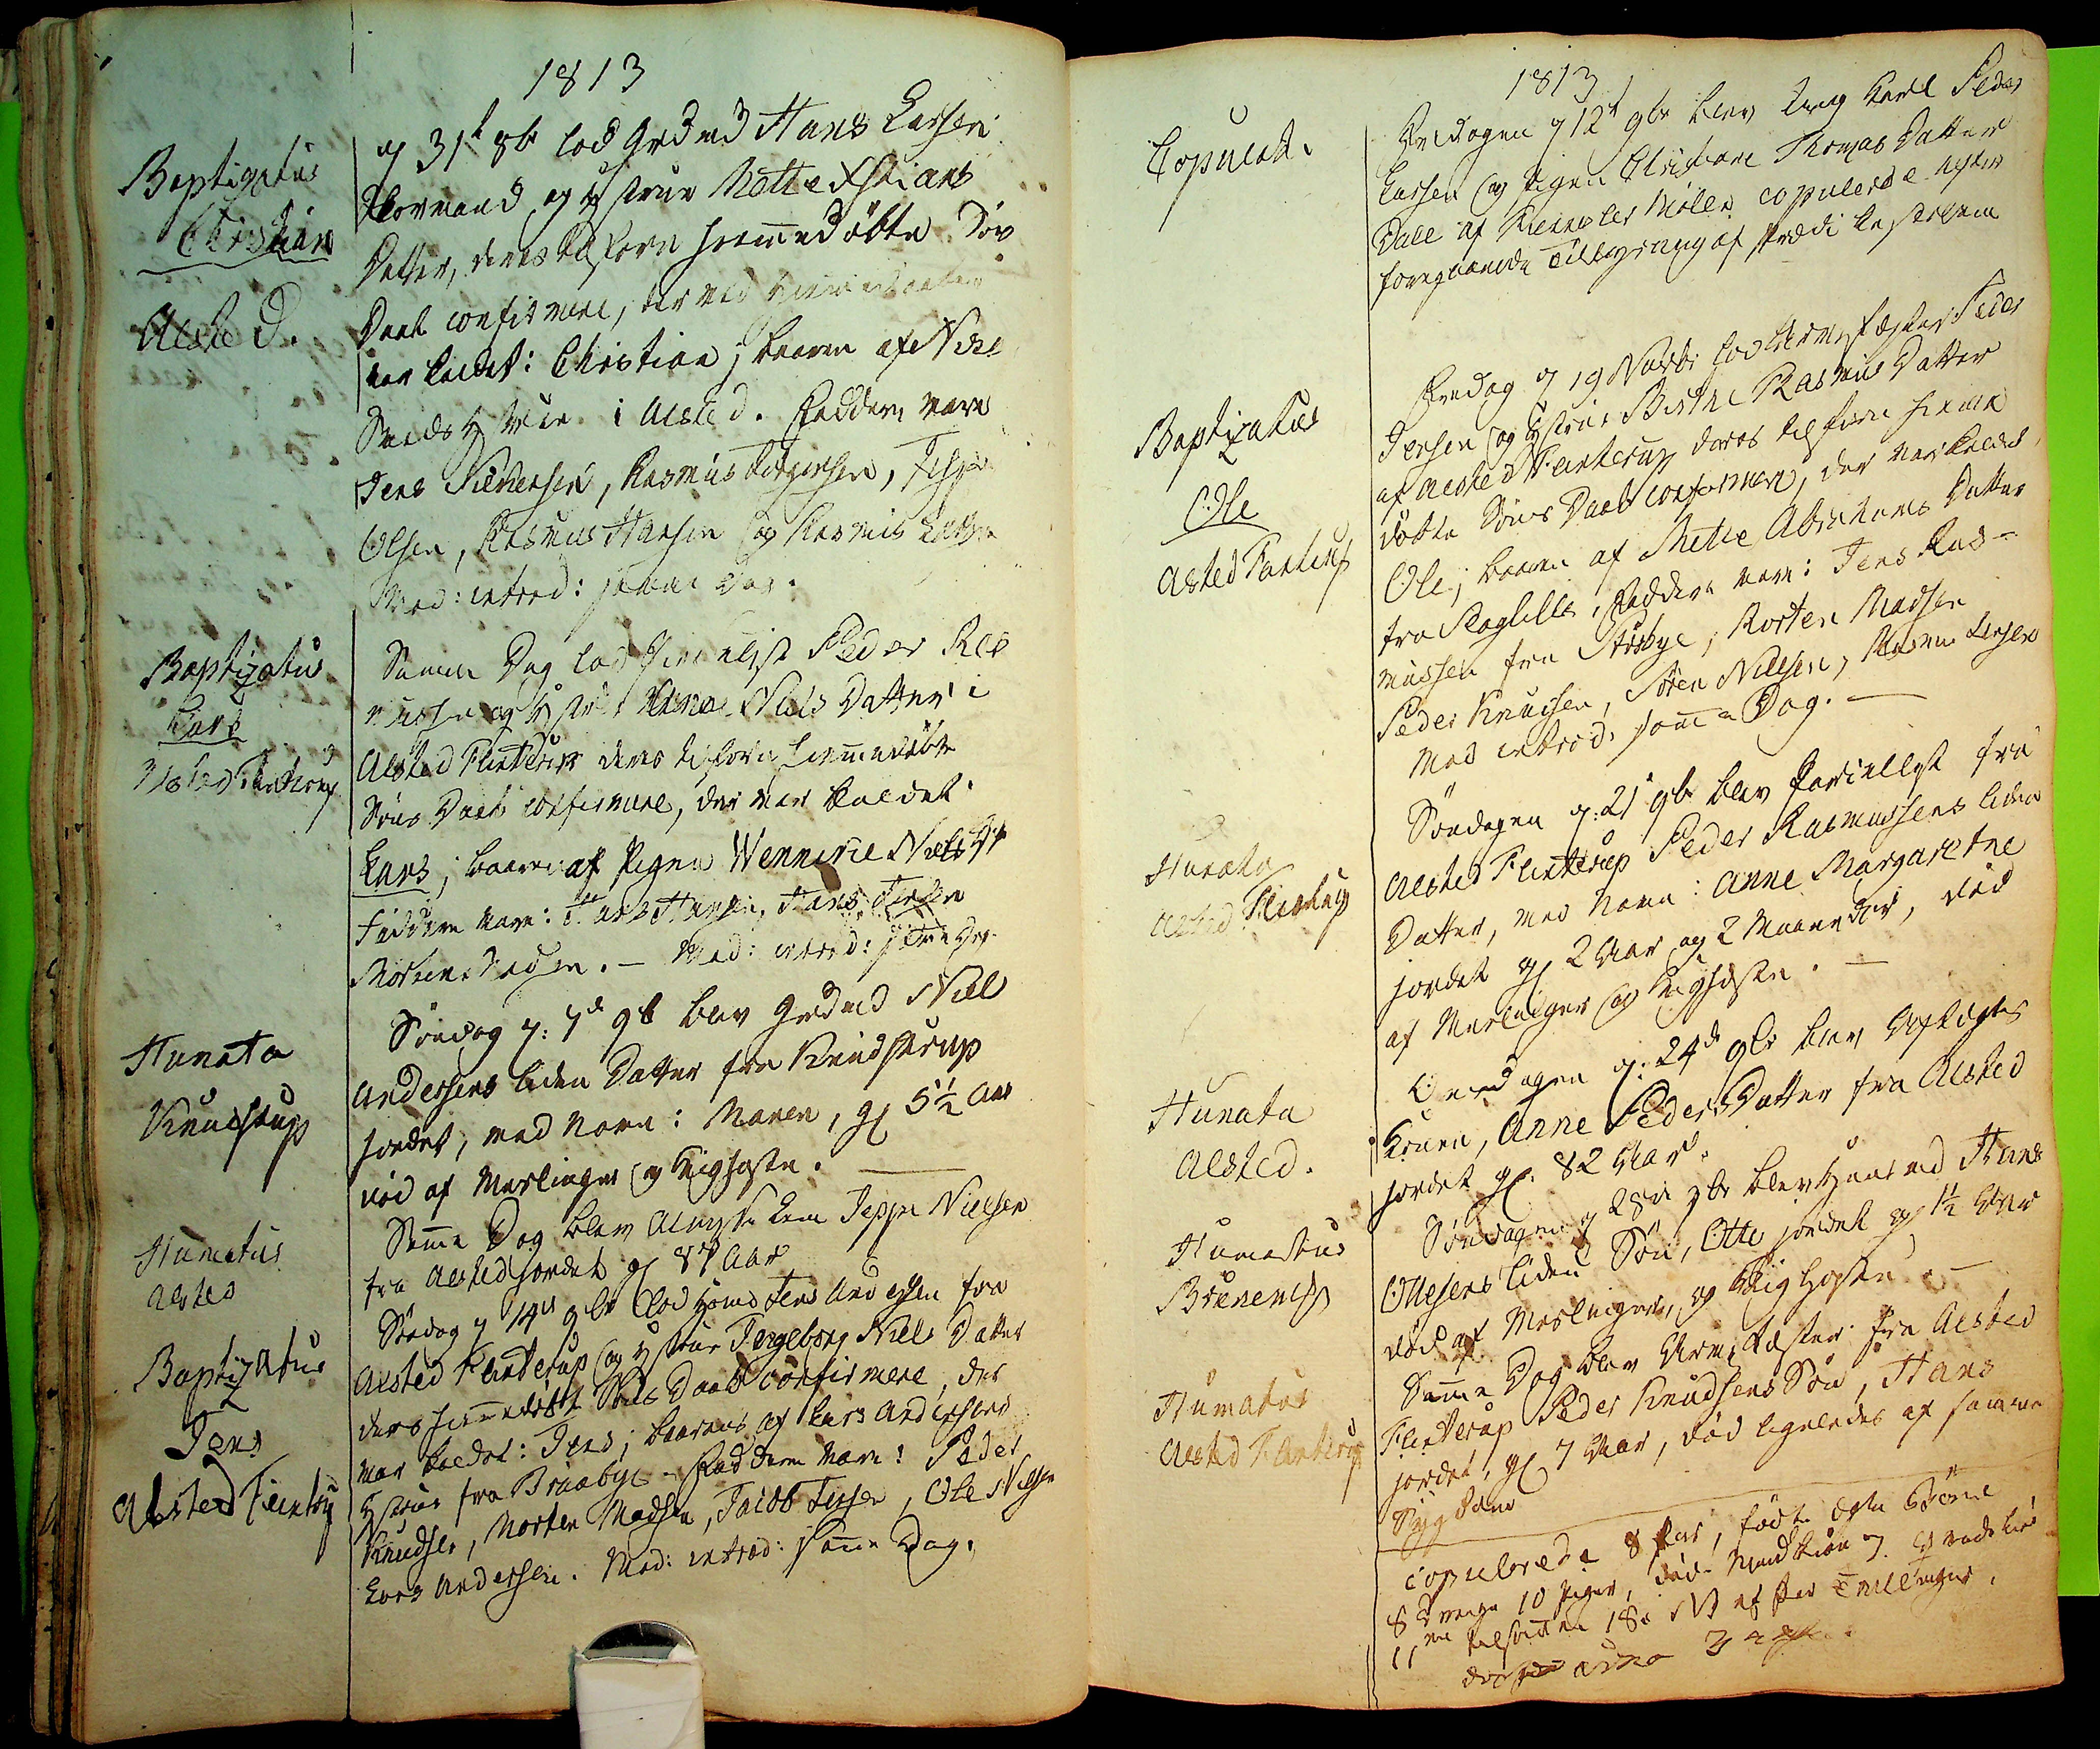Baptizatus
Christian
Alsted.
Baptizatus
Lars
Alsted Flinterup
Humata
Knudstrup
Hamatus
Alsted
Baptizatus
Jens
Alsted Flinterup
1813
d 31te 8br lod Grdmd Hans Larsen
Skovmand og Hstrue Mette Xstians
Datter, deres tilforn hiemmedøbte Søns
Daab confirmere, der ved Hiemmedaaben
var kaldet: Christian; baaren af Niels
Smeds Hstrue i Alsted. Faddere vare
Jens Silkensen, Rasmus Jørgensen, Jesper
Olsen, Rasmus Hansen og Rasmus Larsen
Mod: introd: samme Dag
Samme Dag lod Parcelist Peder Ras¬
missen og Hstrue Anna Niels Datter i
Alsted deres tilforn hiemmedøbte
Søns Daab confirmere, der var kaldet
Lars, baaren af Pigen Wenneke Niels Dtr
Faddere vare: Hans Hansen, Hans Jensen
Morten Madsen. - Mod: introd: samme Dag.
Søndag d: 7de 9br blev Grdmd Niels
Andersens liden Datter fra Knudstrup
jordet, ved Navn: Maren, gl: 5½ Aar
død af Meslingert og Kighoste.
Samme Dag blev Almisse Lem Jeppe Nielsen
fra Alsted jordet gl 87 Aar
Søndag d 14de 9br lod Hsmd Jens Andersen fra
Alsted Flinterup og Hstruee Ingeborg Niels Datter
deres hiemmedøbte Søns Daab conformere, der
var kaldet: Jens; baaren af Lars Andersens
Hstrue fra Braabye. Fadderne vare: Peder
Knudsen, Morten Madsen, Jacob Jensen, Ole Nielsen
Lars Anderssen. Mod: introd: samme Dag.
Copulati
Baptizatus
Ole
Alsted Flinteruo
Humata
Alsted Flinterup
Humata
Alsted
Humatus
Brunem##
Humatus
Alsted Flinterup
1813
Fredagen d 12te 9br blev ung Karl Peder
Larsen, og Pigen Christiane Thomas Datter
Dall af Fienneslev Mølle copulerede efter
foregaaende Tillysning af Prædikestolen
Fredagen d 19 Novbr lod Arvefæster Peder
Jensen og Hstrue Birte Rasmus Datter
af Alsted Flinterup deres tilforn hiemme
døbte Søns Daab confirmere, der var kaldet
Ole; baaren af Mette Abrahams Datter
fra Slaglille, Faddere vare: Jens Ras¬
mussen fra S##bye, Morten Madsen
Peder Knudsen, Søren Nielsen, Rasmus ##sen
Mod introd samme Dag.
Søndagen d: 21de 9br blev Parcelist fra
MarAlsted Flinterup Peder Rasmussens liden
Datter, ved Navn: Anne Margarethe
jordet gl 2 Aar 2 Maaneder, død
Meslinger og Kighoste
Onsdagen d: 24de 9br blev Aftægts
Konen, Anne Perders Datter fra Alsted
jordet gl 82 Aar
Søndagen d 28de 9br blev Huusmd Hans
Ottesens liden Søn, Otte jordet 1½ Aar
død af Meslinger og Kighoste
Samme Dag blev Arvefæster fra Alsted
Flinterup Peder Knudsens Søn, Hans
jordet, gl 7 Aar, død ligeledes af samme
Sygdom
copulere 8 Par, født ægte Børn
8 Drenge 10 Piger, død Mandkiøn 7 Qvindekiøn
11## tilsammen 18. ## ## ## ##
## ## 3 ##
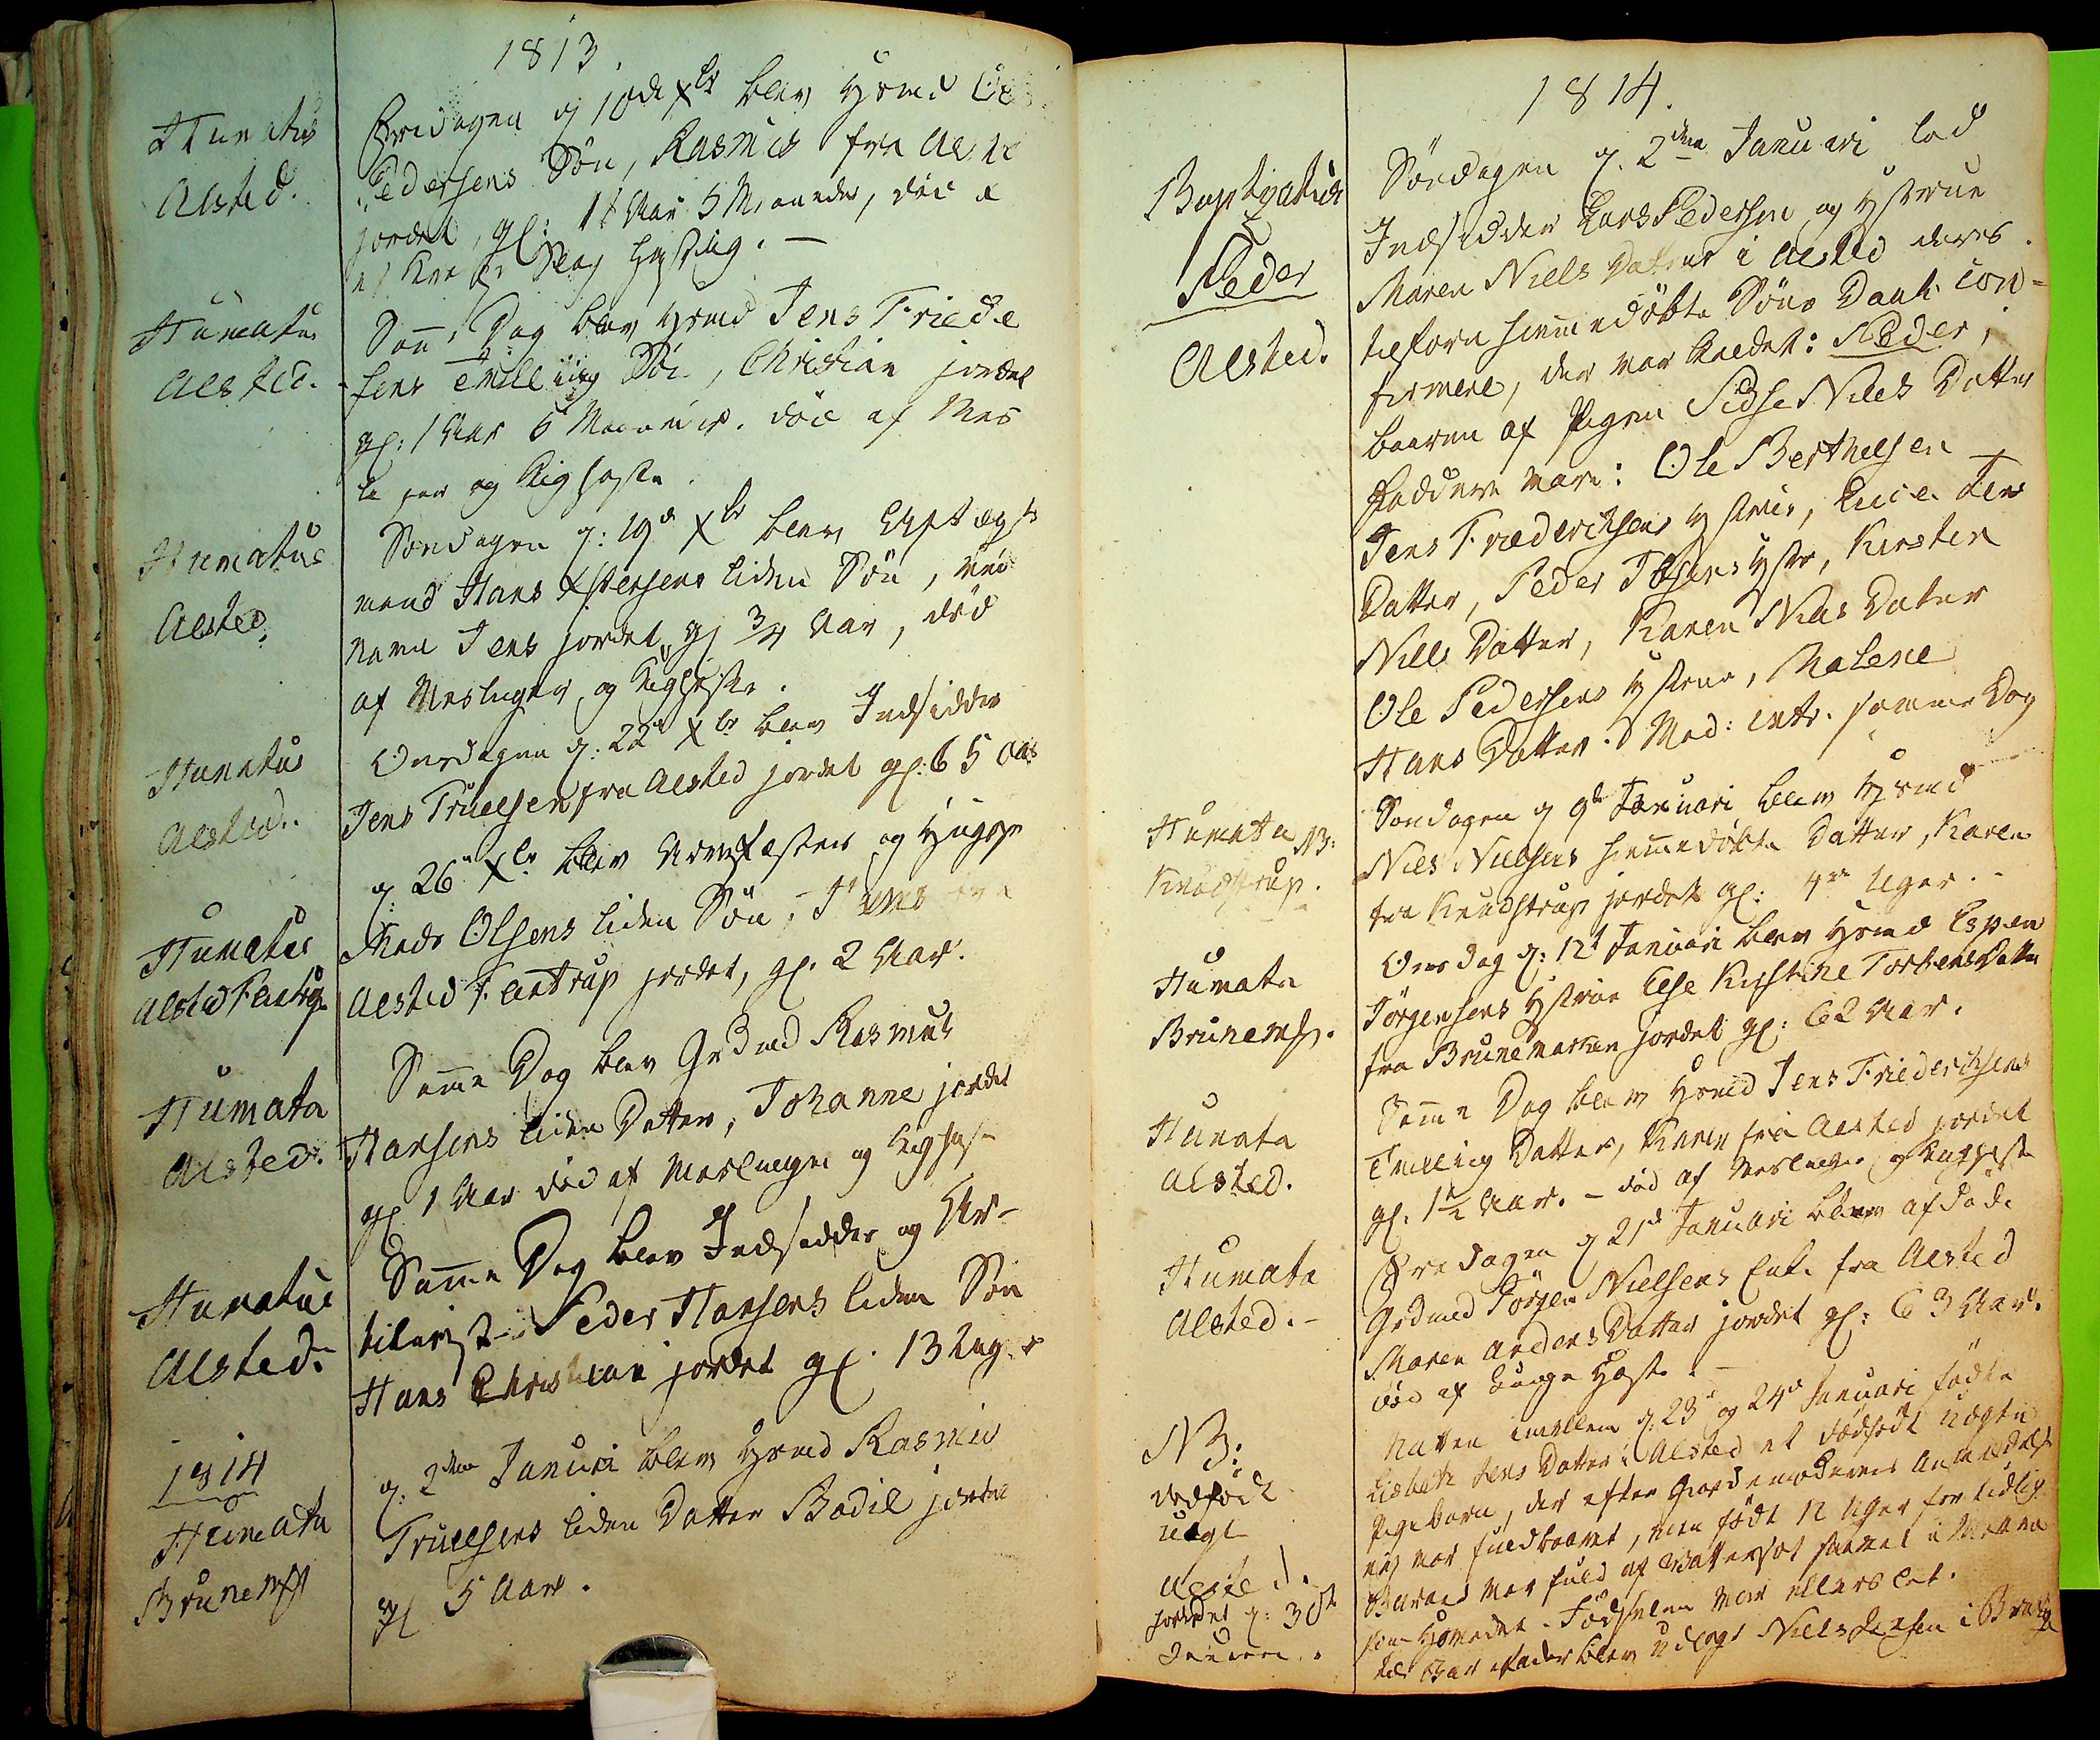Humatus
Alsted.
Humatus
Alsted.
Humatus
Alsted.
Humatus
Alsted
Humatus
Alsted Flinterup
Humata
Alsted.
Humatus
Alsted.
1814
Humata
Brunem##
1813
Fredagen d: 10de Xbr blev Hsmd
Pedersens Søn, Rasmus fra Alsted
Jordet gl: 1 ## aar 5 Maaneder, død i
## ## Slag H## ig
Søn Dag blev Hsmd Jens Friede
sens Tvilling Søn, Christian Jordet
gl: 1 aar 6 Maaneder. død af Mes
linger og Kighoste
Søndagen d: 19de Xbr blev Aftægts
mand Hans Xstersens liden Søn, ved
Navn Jens jordet gl ¾ Aar, død
af Meslinger og Kighoste
Onsdagen d: 22de Xbr blev Indsidder
Jens Truelsen fra Alsted jordet gl: 65 Aar
d: 26de Xbr blev Arvefæster og Hug##
Mads Olsens liden Søn. Jens
Alsted Flinterup jordet, gl: 2 Aar.
Samme Dag bar Grdmd Rasmus
Hansens liden Datter, Johanne jordet
gl 1 Aar død af Meskinger og Kighoste
Samme Dag blev Indsidder og Ar¬
tilerist Peder Hansens liden Søn
Hans Christian jordet gl. 13 Uger
d: 2den Januari blev Hsmd Rasmus
Truelsens liden Datter Bodil jordet
gl 5 Aar.
Baptizatus
Peder
Alsted.
Humata NB:
Knudstrup
Hamata
Brunem##.
Humata
Alsted.
Humata
Alsted.
NB:
dødfødt
Uægte
Uegte
jordet d: 30te
Januari
1814.
Søndagen d: 2den Januari lod
Indsidder Lars Pedersens og Hustrue
Maren Niels Datter i Alsted deres
tilforn hiemmedøbte Søns Daab Con¬
firmere, der var kaldet: Peder,
baaren af Pigen Sidse Niels Datter
Faddere vare: Ole Bertelsen
Jens Friderichsens Hstrue, Licie Jens
Datter, Peder Ibsens Hstr, Kirsten
Niels Datter, Karen Niels Datter
Ole Pedersens Hustrue, Malene
Hans Datter Mad: Mod: intr: samme Dag
Søndagen d 9de Januari blev Hsmd
Niels Nielsens hiemmedøbte Datter, Karen
fra Knudstrup jordet gl: 4## Uger
Onsdag d: 12te Januari blev Hsmd Espen
Jørgensens Hstrue Else Kirstine Torbens Datter
fra Brunemarken jordet gl: 62 Aar.
Samme Dag blev Hsmd Jens Friederichsens
Tvilling Datter, Karen fra Alsted jordet
gl: 1½ Aar. - død af Meslinger og Kighoste
Fredagen d 21de Januari bleve afdøde
Grdmd Jørgen Nielsens Enke fra Alsted
Maren Anders Datter jordet gl: 63 Aar.
død af Lunge Hoste.
Natten imellem d: 23de og 24de Januari fødte
Lisbeth Jens Datter i Alsted et dødfødt uægte
Pigebarn, der efter Giordemoderens Anmeldelse
ey var fuldbaaret, men født 12 Uger for tidlig
Barnet var fuld af Vattersot saaret i M##
## Hovedet. Fødselen var ellers let.
til Barne Fader blev udlagt Niels Jensen i